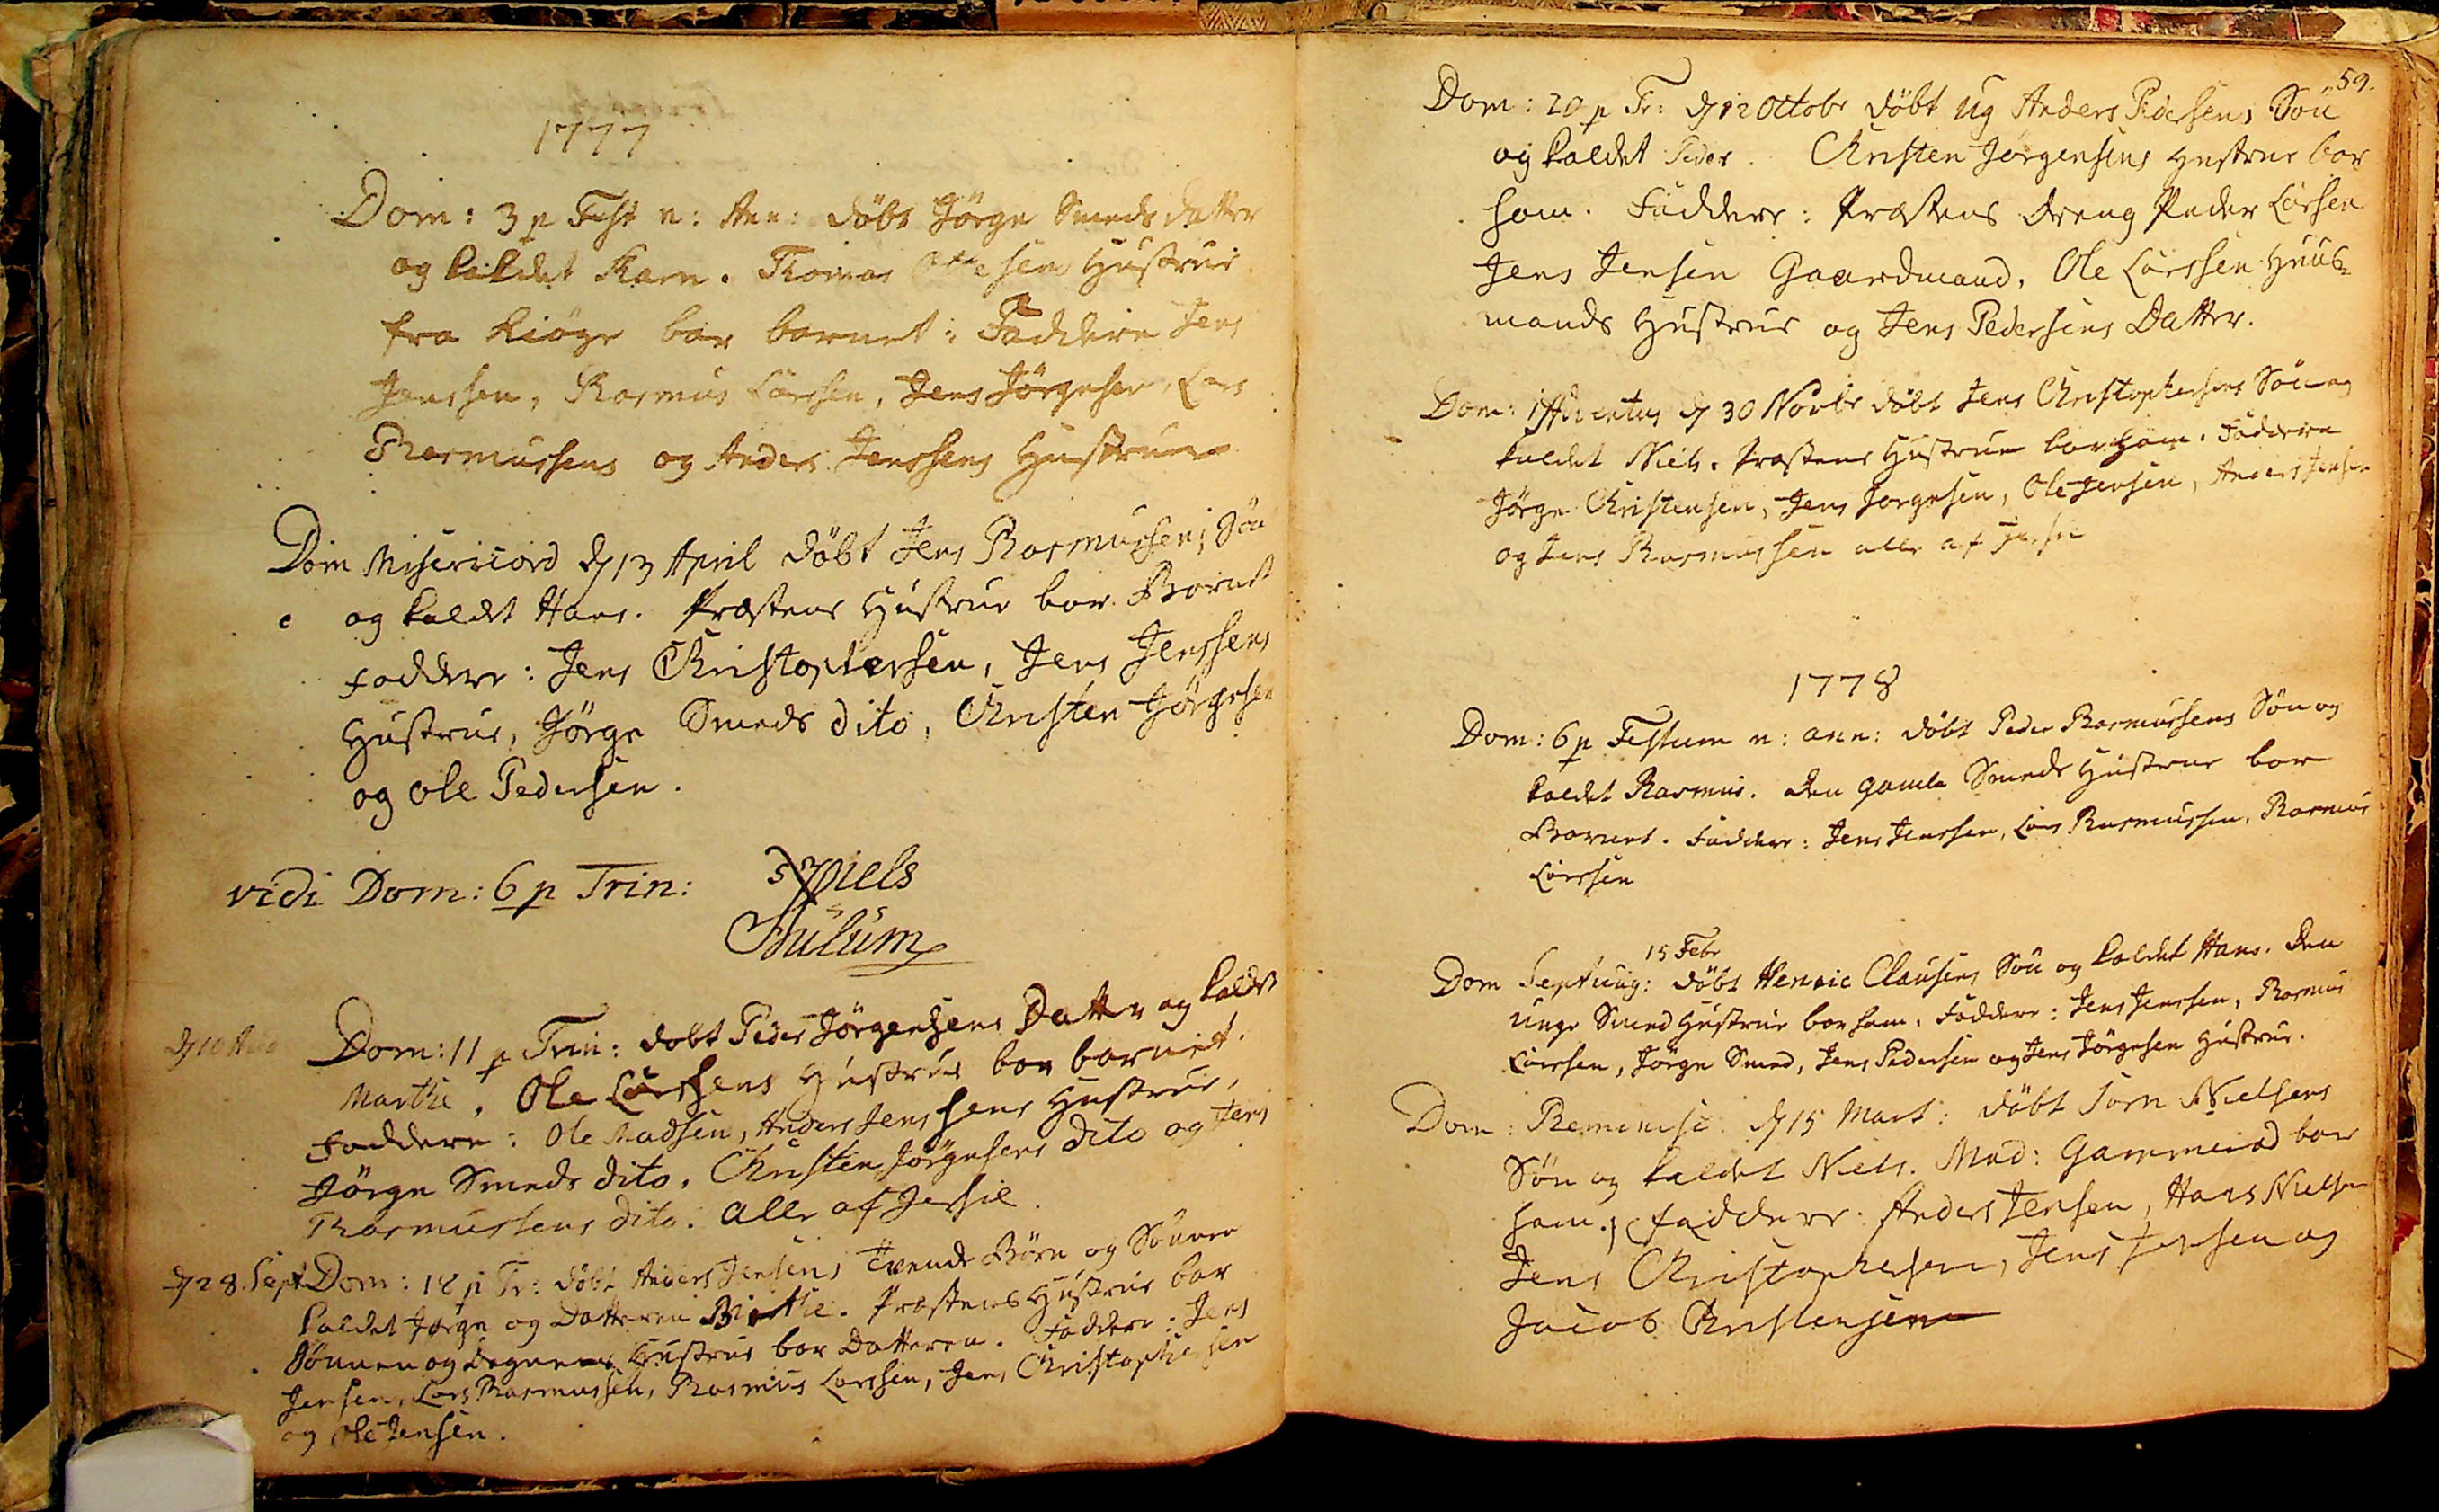1777
Dom: 3 p Fest n: Ann: døbt Jørgn Smeds datter
og kaldet Karn. Thomas Ottesens Hustrue
fra Kiøge bar Barnet: Faddere Jens
Jenssen, Rasmus Larssen, Jens Jørgnsen, Lars
Rasmussen og Anders Jenssens Hustruer.
Dom Misericord d 13 April døbt Jens Rasmussens Søn
og kaldt Hans. Præstens Hustrue bar Barnet
Faddere: Jens Christophersen, Jens Jenssens
Hustrue, Jørgn Smeds dito, Christen Jørgnsen
Ole Pedersen.
Vidi Dom: 6 p Trin:  
Niels
Aulum 
d 10 Aug Dom: 11 p Trin: døbt Peder Jørgensens Datter og kaldt
Marthe. Ole Larssens Hustrue bar Barnet.
Faddere: Ole Madsen, Anders Jenssens Hustrue,
Jørgn Smeds dito. Christen Jørgnsens dito og Jens
Rasmussens dito. Alle af Jersie.
d 28 Sept Dom: 18 p Tr: døbt Anders Jensens Tvende Børn og Sønnen
kaldet Jørgn og Datteren Birthe. Præstens Hustrue bar
Sønnen og Degnens Hustrue bar Datteren. Faddere: Jens
Jensen, Lars Rasmussen, Rasmus Larssen, Jens Christophersen
og Ole Jensen.
59.
Dom: 20 p Tr: d 12 Octobr døbt Ug Anders Pedersens Søn
og kaldet Peder. Christen Jørgensens Hustrue bar
ham. Faddere: Præstens Dreng Peder Larsen
Jens Jensen Gaardmand, Ole Larssen Huus¬
mands Hustrue og Jens Pedersens Datter.
Dom: 1 Adventus d 30 Novbr døbt Jens Christophersens Søn og
kaldet Niels. Præstens Hustrue bar ham. Faddere
Jørgn Christensen, Jens Jørgnsen, Ole Jensen, Anders Jensen
og Lars Rasmussen alle af Jersie.
1778 
Dom: 6 p Festum n: Ann: døbt Peder Rasmussens Søn og
kaldet Rasmus. Den gamle Smeds Hustrue bar 
Barnet. Faddere: Jens Jenssen, Lars Rasmussen, Rasmus
Larssen
15 Febr
Dom: Septuag: døbt Henric Clausens Søn og kaldet Hans. Den
unge Smed Hustrue bar ham. Faddere: Jens Jenssen, Rasmus
Larssen, Jørgen Smed, Jens Pedersen og Jens Jørgnsens Hustrue.
Dom: Reminisc: d 15 Mart: døbt Sørn Nielsens
Søn og kaldet Nils. Mad: Gammerød bar
ham., Faddere: Anders Jensen, Hans Nielsen
Jens Christophersen, Jens Jensen og
Jacob Christensen